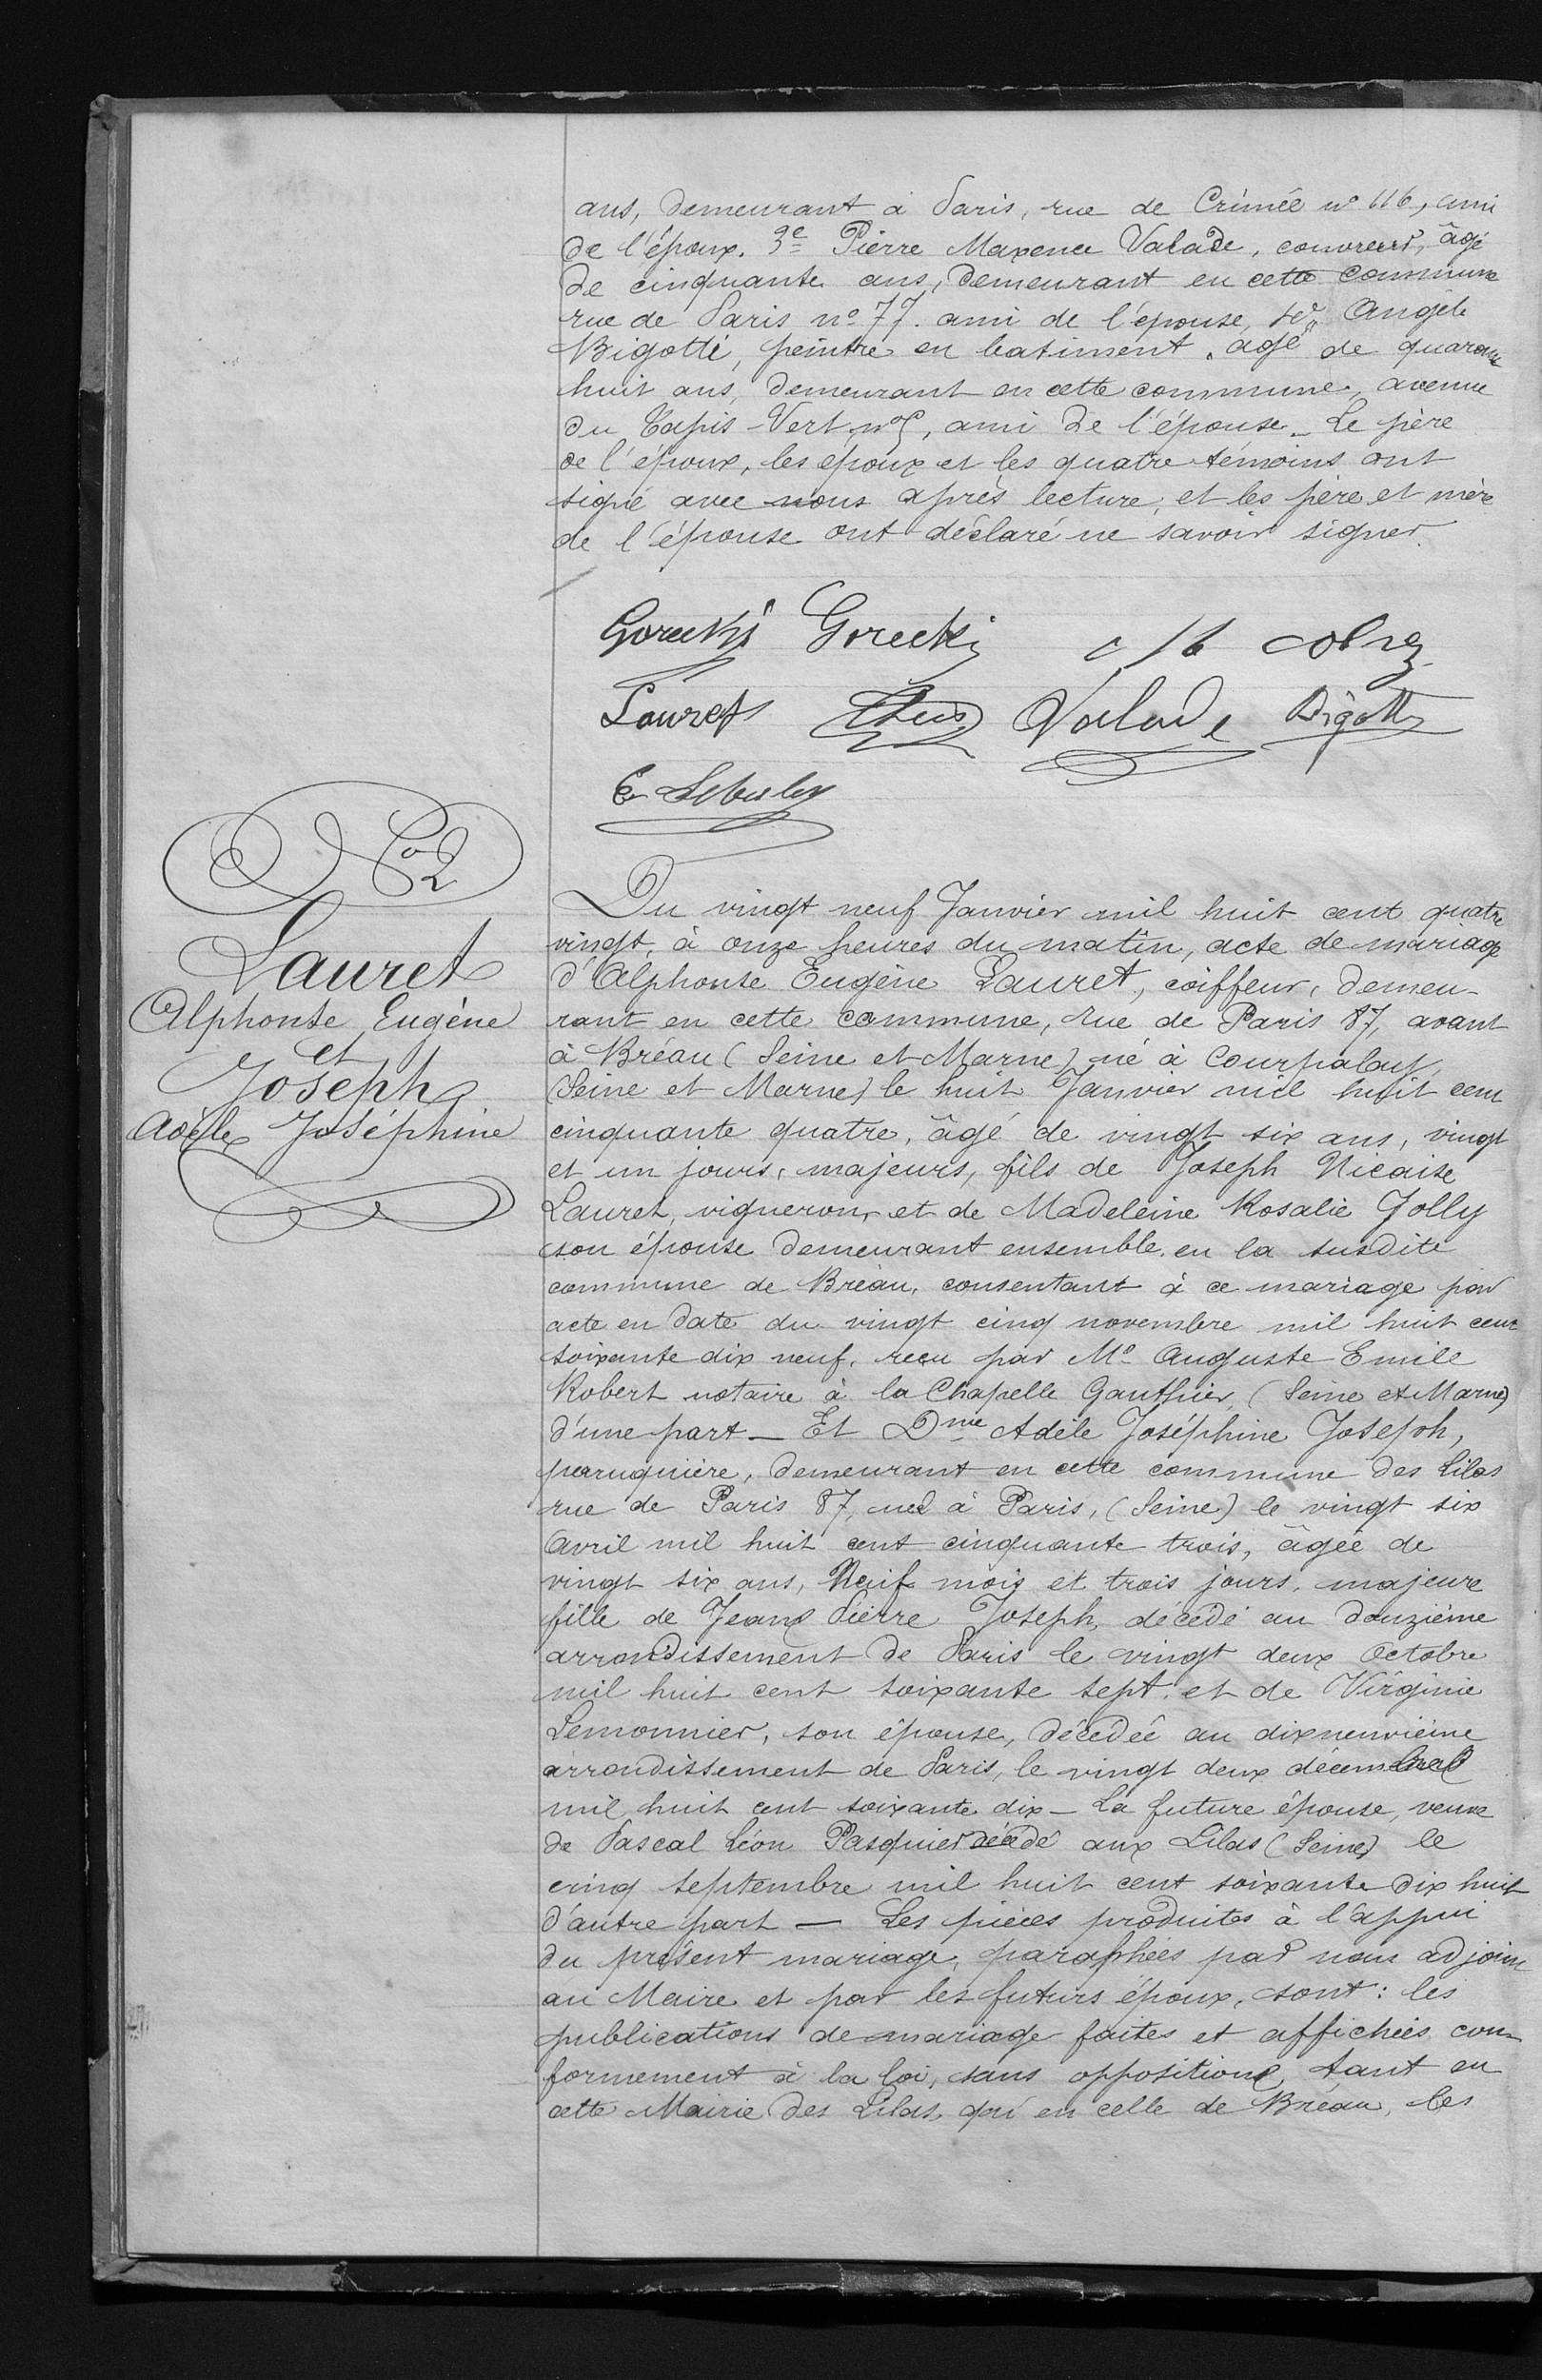ans, demeurant à Paris, rue de Crimée N°116, ami
de l'époux. 3°: Pierre Maxence Valade, couvreur, âgé
de cinquante ans, demeurant en cette commune
rue de Paris N°77 ami de l'épouse, 4° Angèle
Bigotti, peintre en batiment. agé de quarante
huit ans, demeurant en cette commune avenue
du Tapis-Vert N°5, ami de l'épouse. Le père
de l'époux, les époux et les quatre témoins ont
signé avec nous après lecture; et les père et mère
de l'épouse ont déclaré ne savoir signer
N°2
Lauret
Alphonse Eugène
et
Joseph
Adèle Joséphine
Du vingt neuf Janvier mil huit cent quatre
vingt, à onze heures du matin, acte de mariage
d'Alphonse Eugène Lauret, coiffeur, demeu-
rant en cette commune, rue de Paris 87, avant
à Bréau (Seine et Marne) né à Courpaloup
(Seine et Marne) le huit Janvier mil huit cent
cinquante quatre, âgé de vingt-six ans, vingt
et un jours, majeurs, fils de Joseph Nicaise
Lauret, vigneron, et de Madeleine Rosalie Jolly
son épouse demeurant ensemble en la susdite
commune de Bréan, consentant à ce mariage par
acte en date du vingt cinq novembre mil huit cent
soixante dix neuf, reçu par M°-Auguste Emile
Robert notaire à la Chapelle Gauthier (Seine et Marne)
d'une part-Et Dme Adèle Joséphine Joseph
perruquière, demeurant en cette commune des lilas
rue de Paris 87, née à Paris, (Seine) le vingt six
avril mil huit cent cinquante trois, âgée de
vingt six ans, neuf mois et trois jours, majeure
fille de Jean Pierre Joseph, décédé au douzième
arrondissement de Paris le vingt deux octobre
mil huit cent soixante sept et de Virginie
Lemonnier, son épouse, décédée au dix neuvième
arrondissement de Paris, le vingt deux décembre
mil huit cent soixante dix-La future épouse, veuve
de Pascal Léon Pasquier décédé aux Lilas (Seine) le
cinq septembre mil huit cent soixante dix huit
d'autre part -Les pièces produites à l'appui
du présent mariage paraphées par nous adjoint
au Maire et par les futurs époux, sont: les
publications de mariage faites et affichées con
formément à la loi, sans opposition tant en
cette Mairie des Lilas, qui en celle de Bréau, les
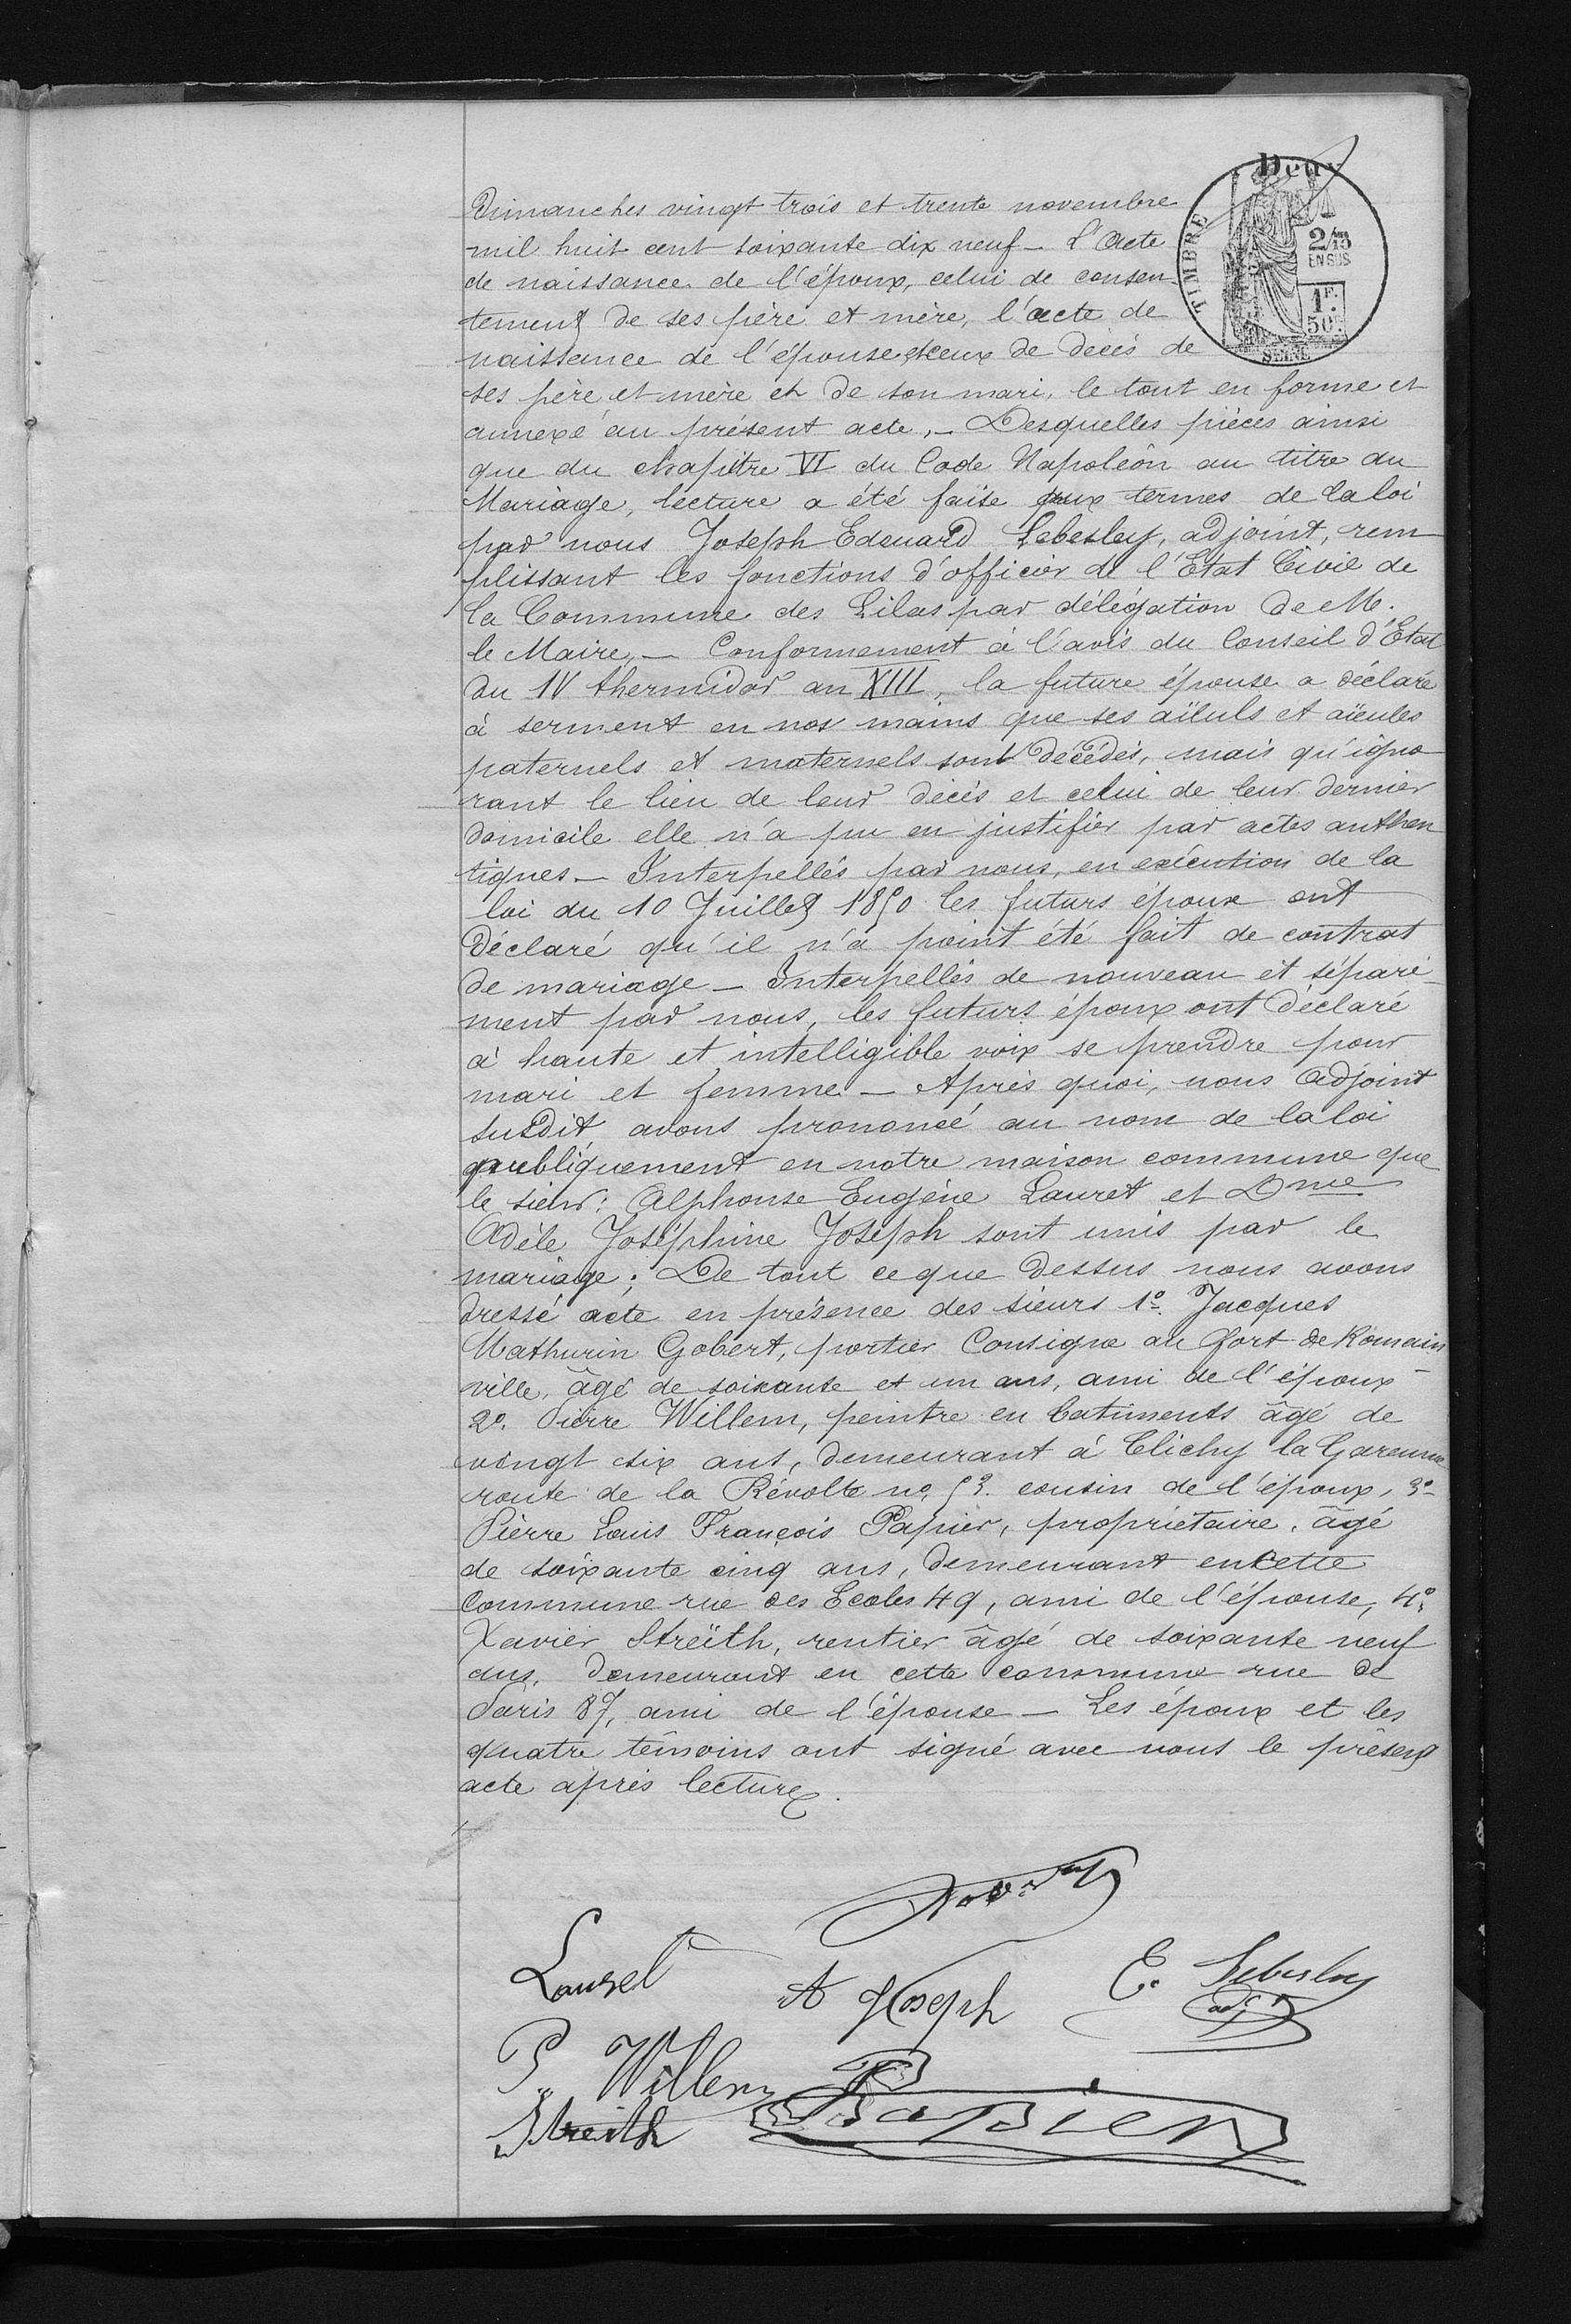Dimanches vingt trois et trente novembre
mil huit cent soixante dix neuf. l'acte
de naissance de l'époux, celui de consen
tement de ses père et mère, l'acte de
naissance de l'épouse et ceux de décès de
ses père et mère et de son mari le tout en forme et
annexé au présent acte,-Desquelles pièces ainsi
que du chapitre VI du Code Napoléon au titre du
Mariage, lecture a été faite aux termes de la loi
par nous Joseph Edouard Leberley, adjoint, rem
plissant les fonctions d'officier de l'Etat Civil de
la Commune des Lilas par délégation de cette
le Maie -Conformement à l'avis du Conseil d'Etat
du IV thermidor an XIII, la future épouse a déclaré
à serment en nos mains que ses aïeuls et aïeules
paternels et maternels sont décédés, mais qu'igno-
rant le lieu de leur décès et celui de leur dernier
domicile elle n'a pu en justifier par actes authen-
tiques-Interpellés par nous, en exécution de la
loi du 10 Juillet 1850 les futurs époux ont
déclaré qu'il n'a point été fait de contrat
de mariage è Interpellés de nouveau et séparé-
ment par nous, les futurs époux ont déclaré
à haute et intelligible voix se prendre pour
mari et femme -Après quoi, nous adjoint
susdit, avons prononcé au nom de la loi
publiquement en notre maison commune que
le sieur: Alphonse Eugène Lauret et Dme
Adèle Joséphine Joseph sont unis par le
mariage; De tout ce que dessus nous avons
dressé acte en présence des sieurs 1°-Jacques
Mathurin Gobert, portier consigne au fort de Romain
ville âgé de soixante et un ans, ami de l'époux
2°. Pierre Willem, peintre en batiments âgé de
vingt six ans, demeurant à Clichy la Garenne
route de la Révolte N°53 cousin de l'époux, 3°-
Pierre Louis François Papier, propriétaire, âgé
de soixante cinq ans, demeurant en cette
commune rue des Ecoles 49, ami de l'épouse, 4°,
Xavier Streitj, rentier âgé de soixante neuf
ans, demeurant en cette commune rue de
Paris 87, ami de l'épouse -Les époux et les
quatre témoins ont signé avec nous le présent
acte après lecture.